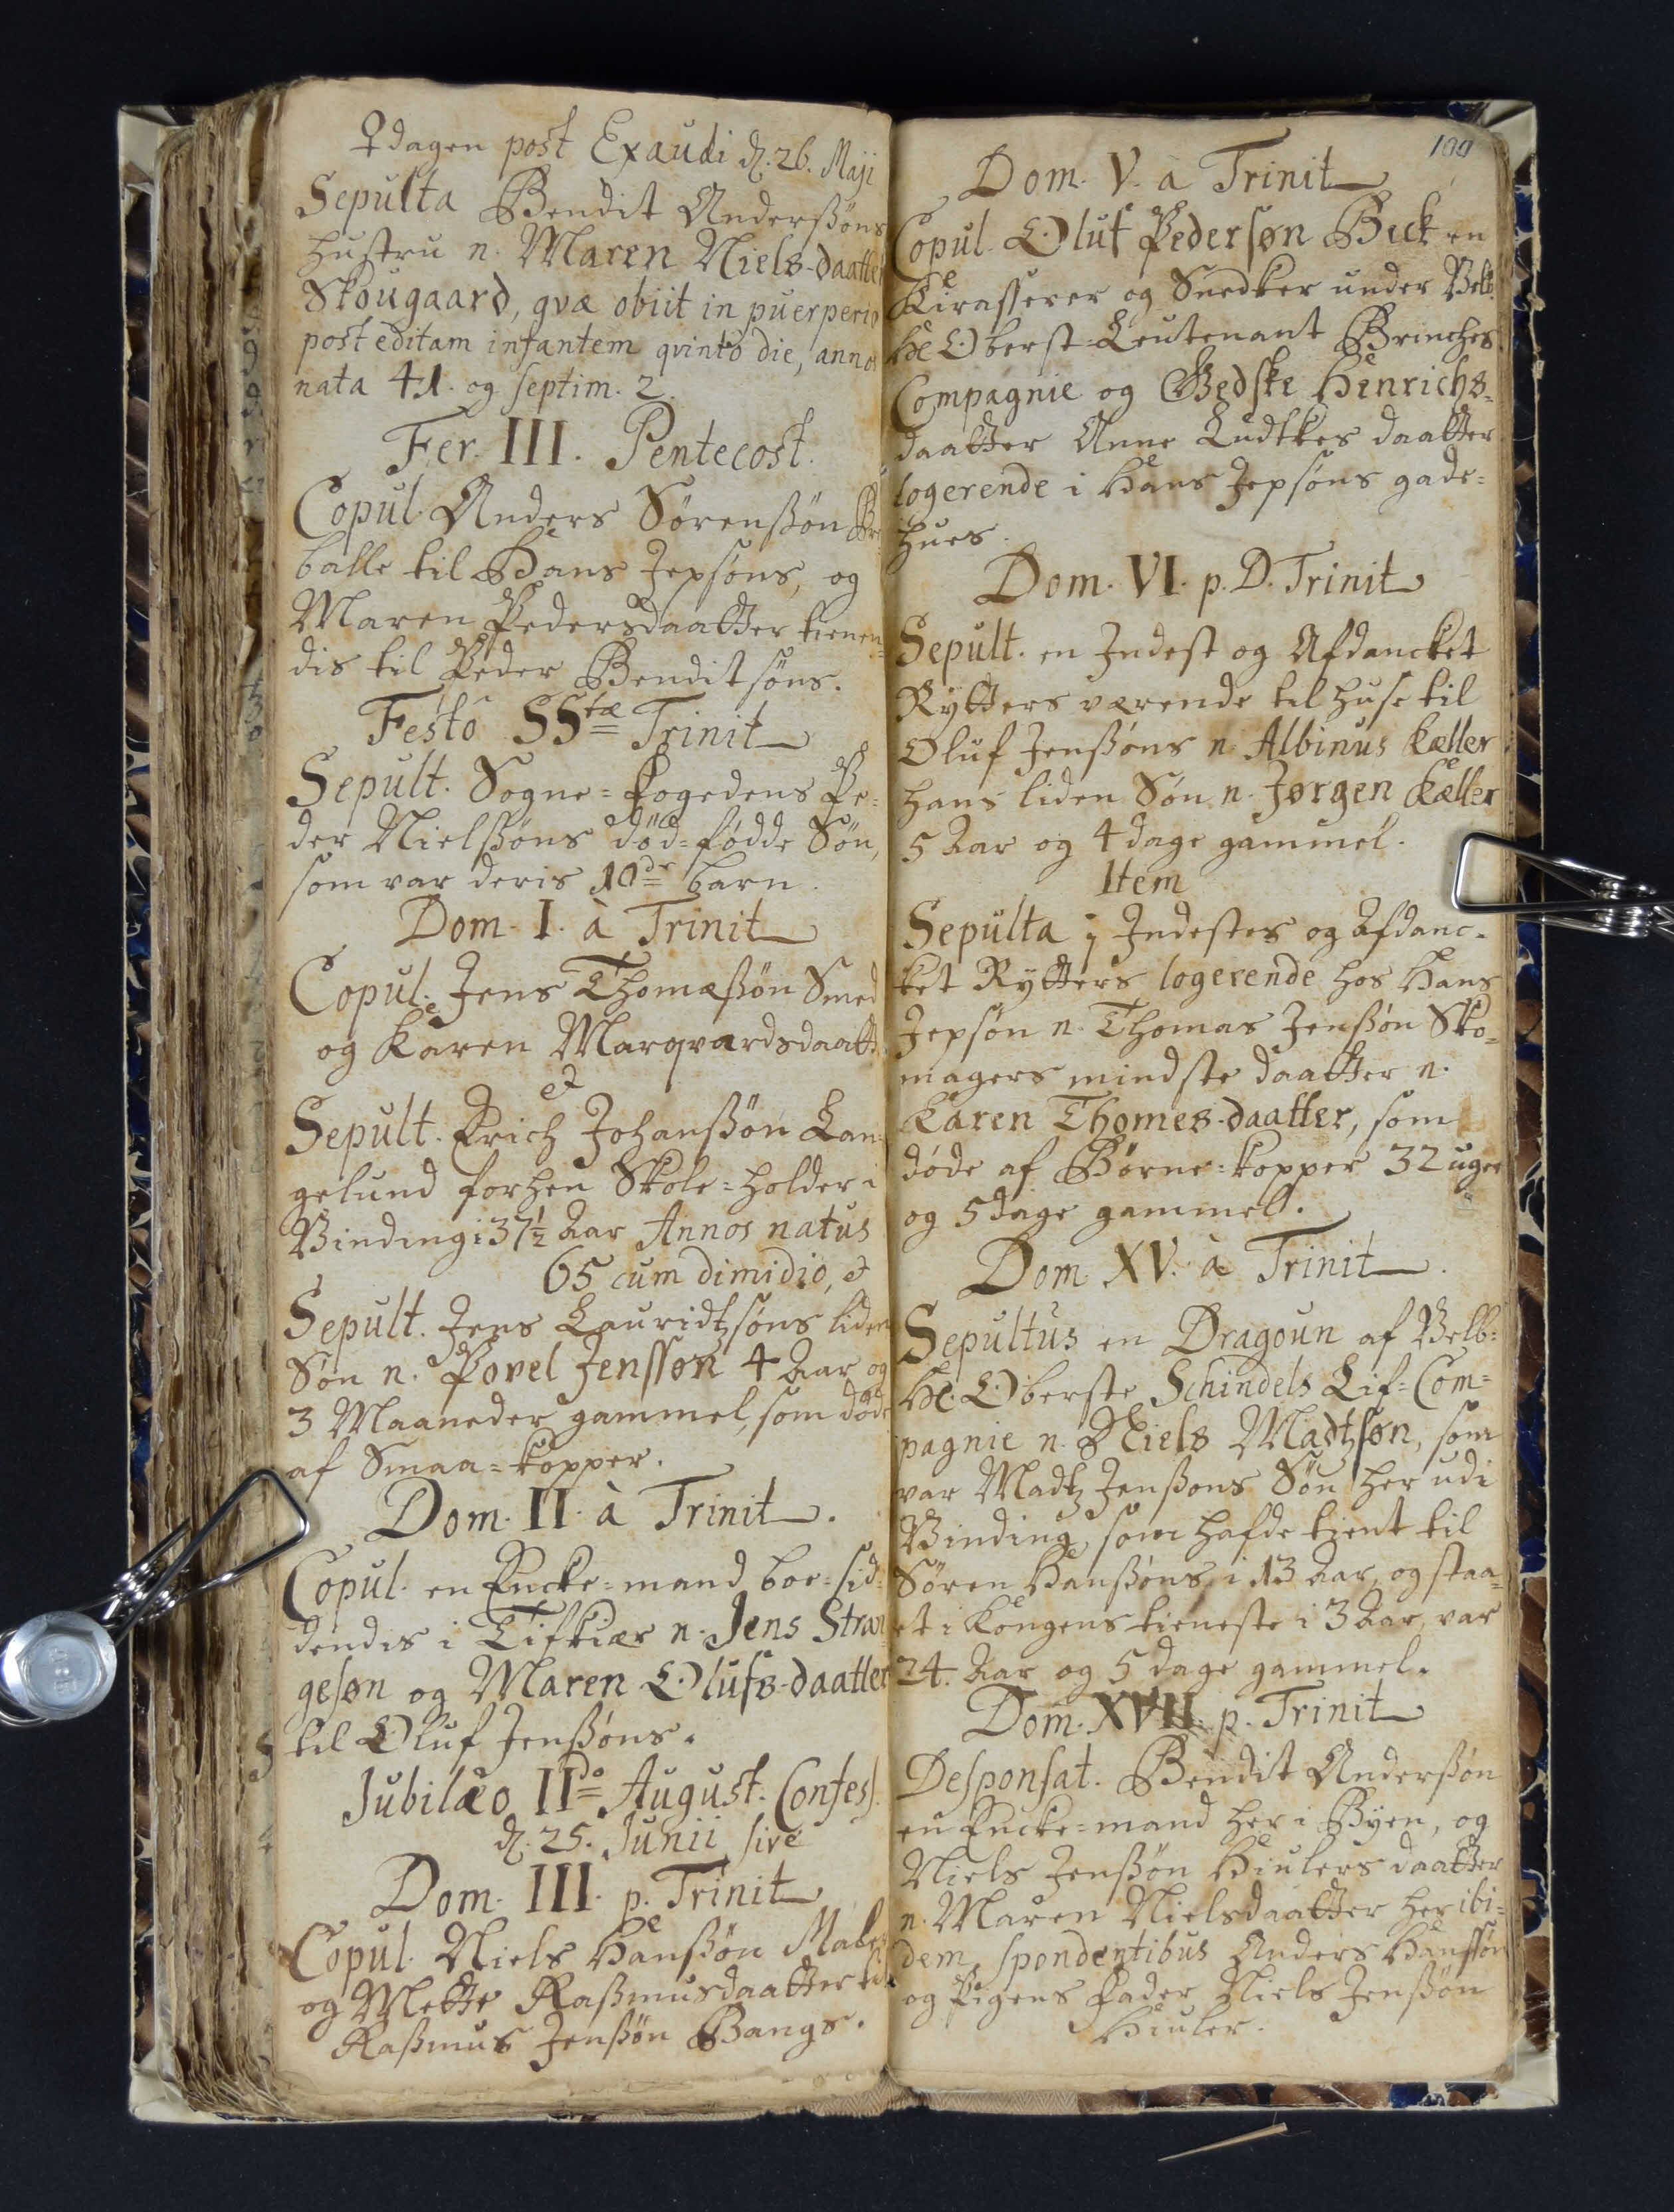☿ dagen post Exaudi d. 26. Maji
Sepulta Bendit Anderssøns
hustru n. Maren Niels-daatter
Skougaard, qvæ obiit in puerperio 
post editam infantem qvinto die, anno  
nata 41 og septim. 2
Fer. III. Pentecost.
Copul. Anders Sørenssøn Bre¬
balle til Hans Jepsøns, og
Maren Pedersdaatter tienen¬
dis til Peder Benditsøns.
Festo SStæ Trinit
Sepult. Sogne=Fogedens Pe¬
der Nielssøns død=fødde Søn,
som var deris 10de barn
Dom. I. a Trinit
Copul. Jens Thomæssøn Smed
og Karen Marqvardsdaatt  
et
Sepult. Erich Johanssøn Lan¬
gelund forhen Skole=holder i
Vinding i 37½ Aar Annos natus
65 cum dimidio, et
Sepult. Jens Lauridtzsøns liden
Søn n. Povel Jenssøn 4 Aar og
3 Maaneder gammel, som døde  
af Smaa=kopper.
Dom. II. a Trinit
Copul. en Encke=mand boe=sid¬
dendis i Tifkiær n. Jens Stran¬ 
gesøn og Maren Olufs-daatte  
til Oluf Jenssøns.
Jubilao IIdo August. Contest.
d. 25. Junii sive
Dom. III p. Trinit
Copul. Niels Hanssøn Maler  
og Mette Rassmusdaatter ti  
Rassmus Jenssøn Bangs.
100
Dom. V. a Trinit
Copul. Oluf Pedersøn Beck en
Kirasserer og Snedker under Velb.
Hl Oberst=Leutenant Brinches
Compagnie og Gedske Henrichs¬
daatter Anne Ludtkes daatter
logerende i Hans Jepsøns gade¬
hues
Dom. VI. p. D. Trinit
Sepult en Indest og Afdancket
Rytters værende til huse til
Oluf Jenssøns n. Albinus Kæller
hans liden Søn n. Jørgen Kæller
5 Aar og 4 dage gammel.
Item
Sepulta 1 Indestes og Afdanc¬
ket Rytters logerende hos Hans 
Jepsøn n. Thomas Jenssøn Sko¬
magers mindste daatter n.
Karen Thomas-daatter, som
døde af Børne=kopper 32 uger
og 5 dage gammel.
Dom. XV. a Trinit
Sepultus en Dragoun af Velb:
Hl Oberste Schindels Lif=Com¬
pagnie n. Niels Madtzsøn, som
var Madtz Jenssøns Søn her udi
Vinding, som hafde tient til
Søren Hanssøns i 13 Aar, og staa¬
et i Kongens tieneste i 3 Aar, var 
24 Aar og 5 dage gammel.
Dom. XVII. p. Trinit
Desponsat. Bendit Anderssøn
en Encke=mand her i Byen, og
Niels Jenssøn Hiulers daatter
n. Maren Nielsdaatter her ibi¬
dem Spondentibus Anders Hanssøn
og Pigens Fader Niels Jenssøn
Hiuler
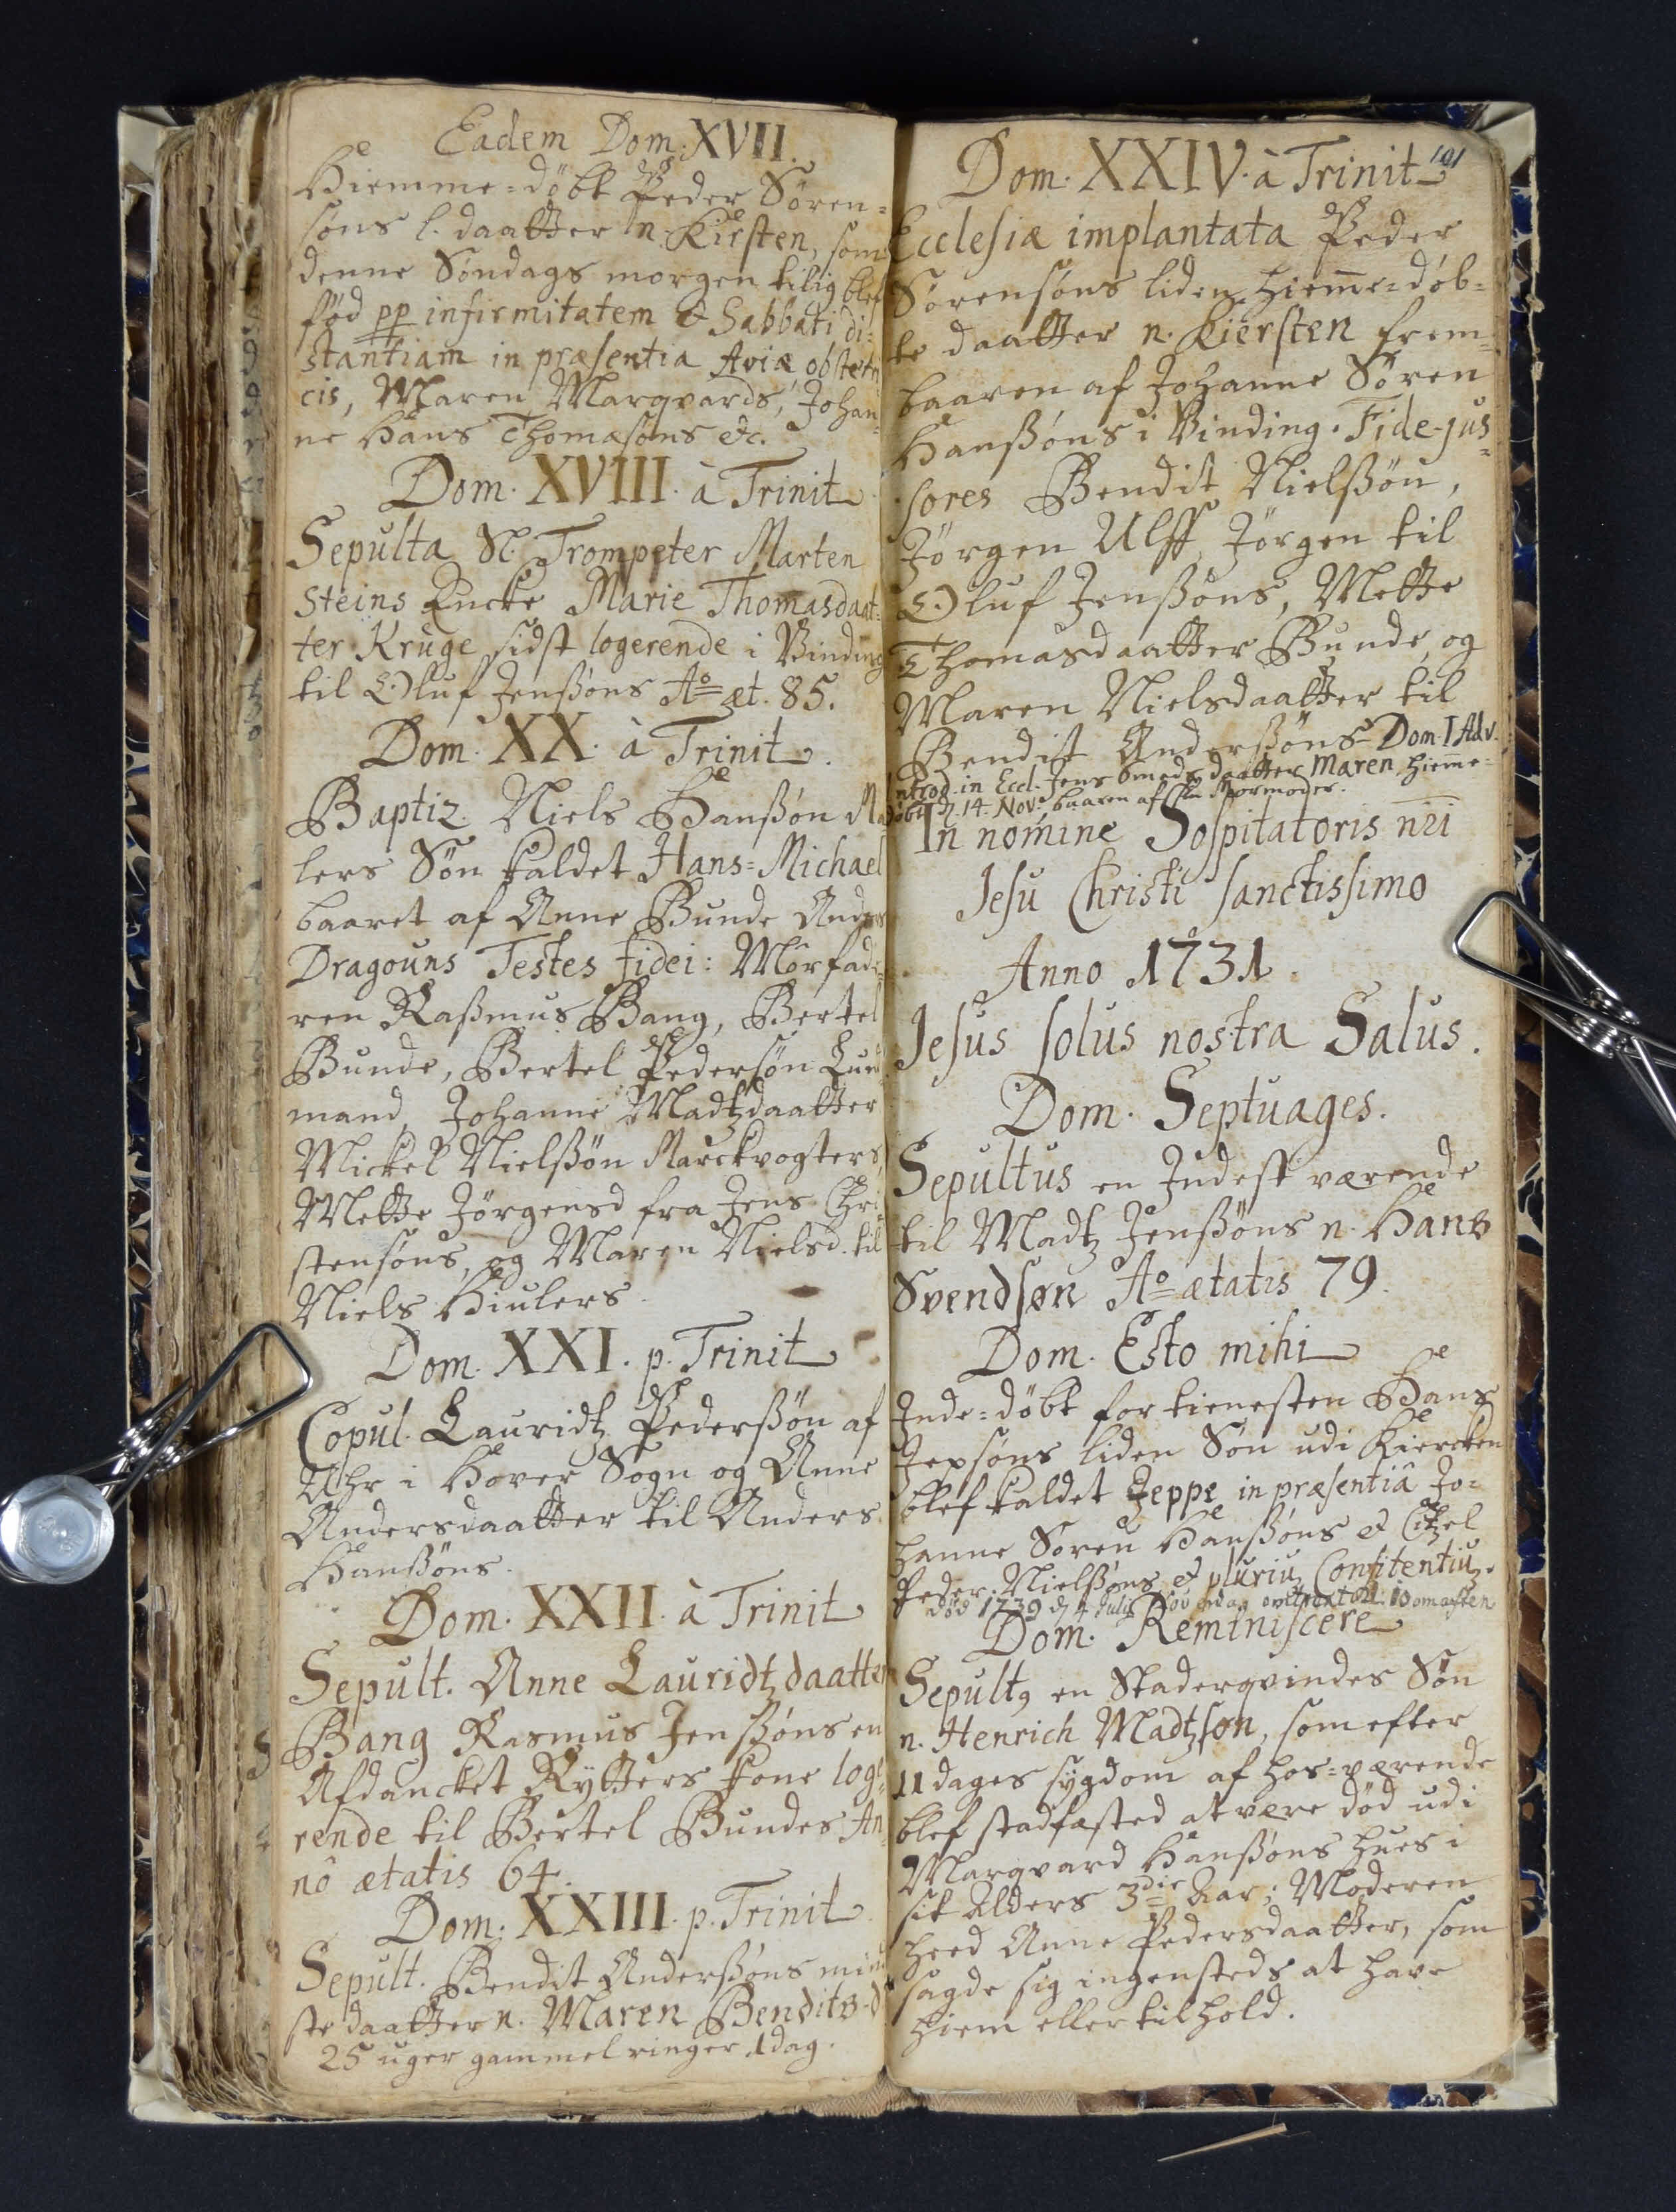Eadem Dom XVII.
Hiemme=døbt Peder Søren¬
søns l. daatter n. Kirsten, som
denne Søndags morgen tidlig blef
fød pp infirmitatem et Sabbati di¬
stantiam in præsentia Aviæ obstetri¬
cis, Maren Marqvards, Johan¬
ne Hans Thomæsøns etc.
Dom. XVIII. a Trinit
Sepulta Sl. Trompeter Marten
Steins Encke Marie Thomasdaat¬
ter Kruge sidst logerende i Vinding
til Oluf Jenssøns Ao æt. 85.
Dom. XX. a Trinit.
Baptiz. Niels Hanssøn Ma¬  
lers Søn kaldet Hans=Michael
baaret af Anne Bunde Anders
Dragouns Teastes fidei: Morfade¬
ren Rasmus Bang, Bertel
Bunde, Bertel Pedersøn Lund¬
mand. Johanne Madtzdaatter
Mickel Nielssøn Marckvogters,
Mette Jørgensd fra Jens Chri¬
stensøns og Maren Nielsd. til
Niels Hiulers
Dom. XXI. p. Trinit
Copul. Lauridtz Pederssøn af
Uhr i Hover Sogn og Anne
Andersdaatter til Anders
Hanssøns
Dom. XXII. a Trinit
Sepult. Anne Lauridtzdaatter
Bang Rasmus Jenssøns en
Afdancket Rytters Kone loge¬
rende til Bertel Bundes An¬
no ætatis 64
Dom. XXIII. p. Trinit
Sepult. Bendit Anderssøns mind  
ste daatter Maren Bendits-d
25 uger gammel ringer 1 dag
101
Dom. XXIV. a Trinit
Ecclesiæ implantata Peder
Sørensøns liden hiemme=døb¬
te daatter n. Kiersten, frem¬
baaren af Johanne Søren
Hanssøns i Vinding. Fide-jus¬
sores Bendit Nielssøn
Jørgen Ulff, Jørgen til
Oluf Jenssøns, Mette
Thomasdaatter Bunde, og
Maren Nielsdaatter til
Bendit Anderssøns.Dom- I Adv.
Introd. in Eccl. Jens Smeds daatter Maren, hiemme¬
døbt d. 14. Nov. baaren af sin Mormoder.
In nomine Sospitatoris
Jesu Christi sanctissimo
Anno 1731
Jesus solus nostra Salus.
Dom. Septuages.
Sepultus en Indest værende
til Madtz Jenssøns n. Hans
Svendsøn Ao ætatis 79
Dom. Esto mihi
Inde=døbt for tienesten Hans
Jepsøns liden Søn udi Kiercken
blef kaldet Jeppe, in præsentia Jo¬
hanne Søren Hanssøns et Citzel
Peder Nielssøns et pluriu Confitentiu.
død 1739 d 4 Julii løverdag## omtrent kl: 10 om aften
Dom. Reminiscere
Sepultq en Staderqvindes Søn
n. Henrich Madtzsøn, som efter
11 dages sygdom af hos=værende
blef stadfæsted at være død udi
Marqvard Hanssøns hues i
sit Alders 3die Aar; Moderen
heed Anne Pedersdaatter, som
sagde sig ingensteds at have
hiem eller tilhold.